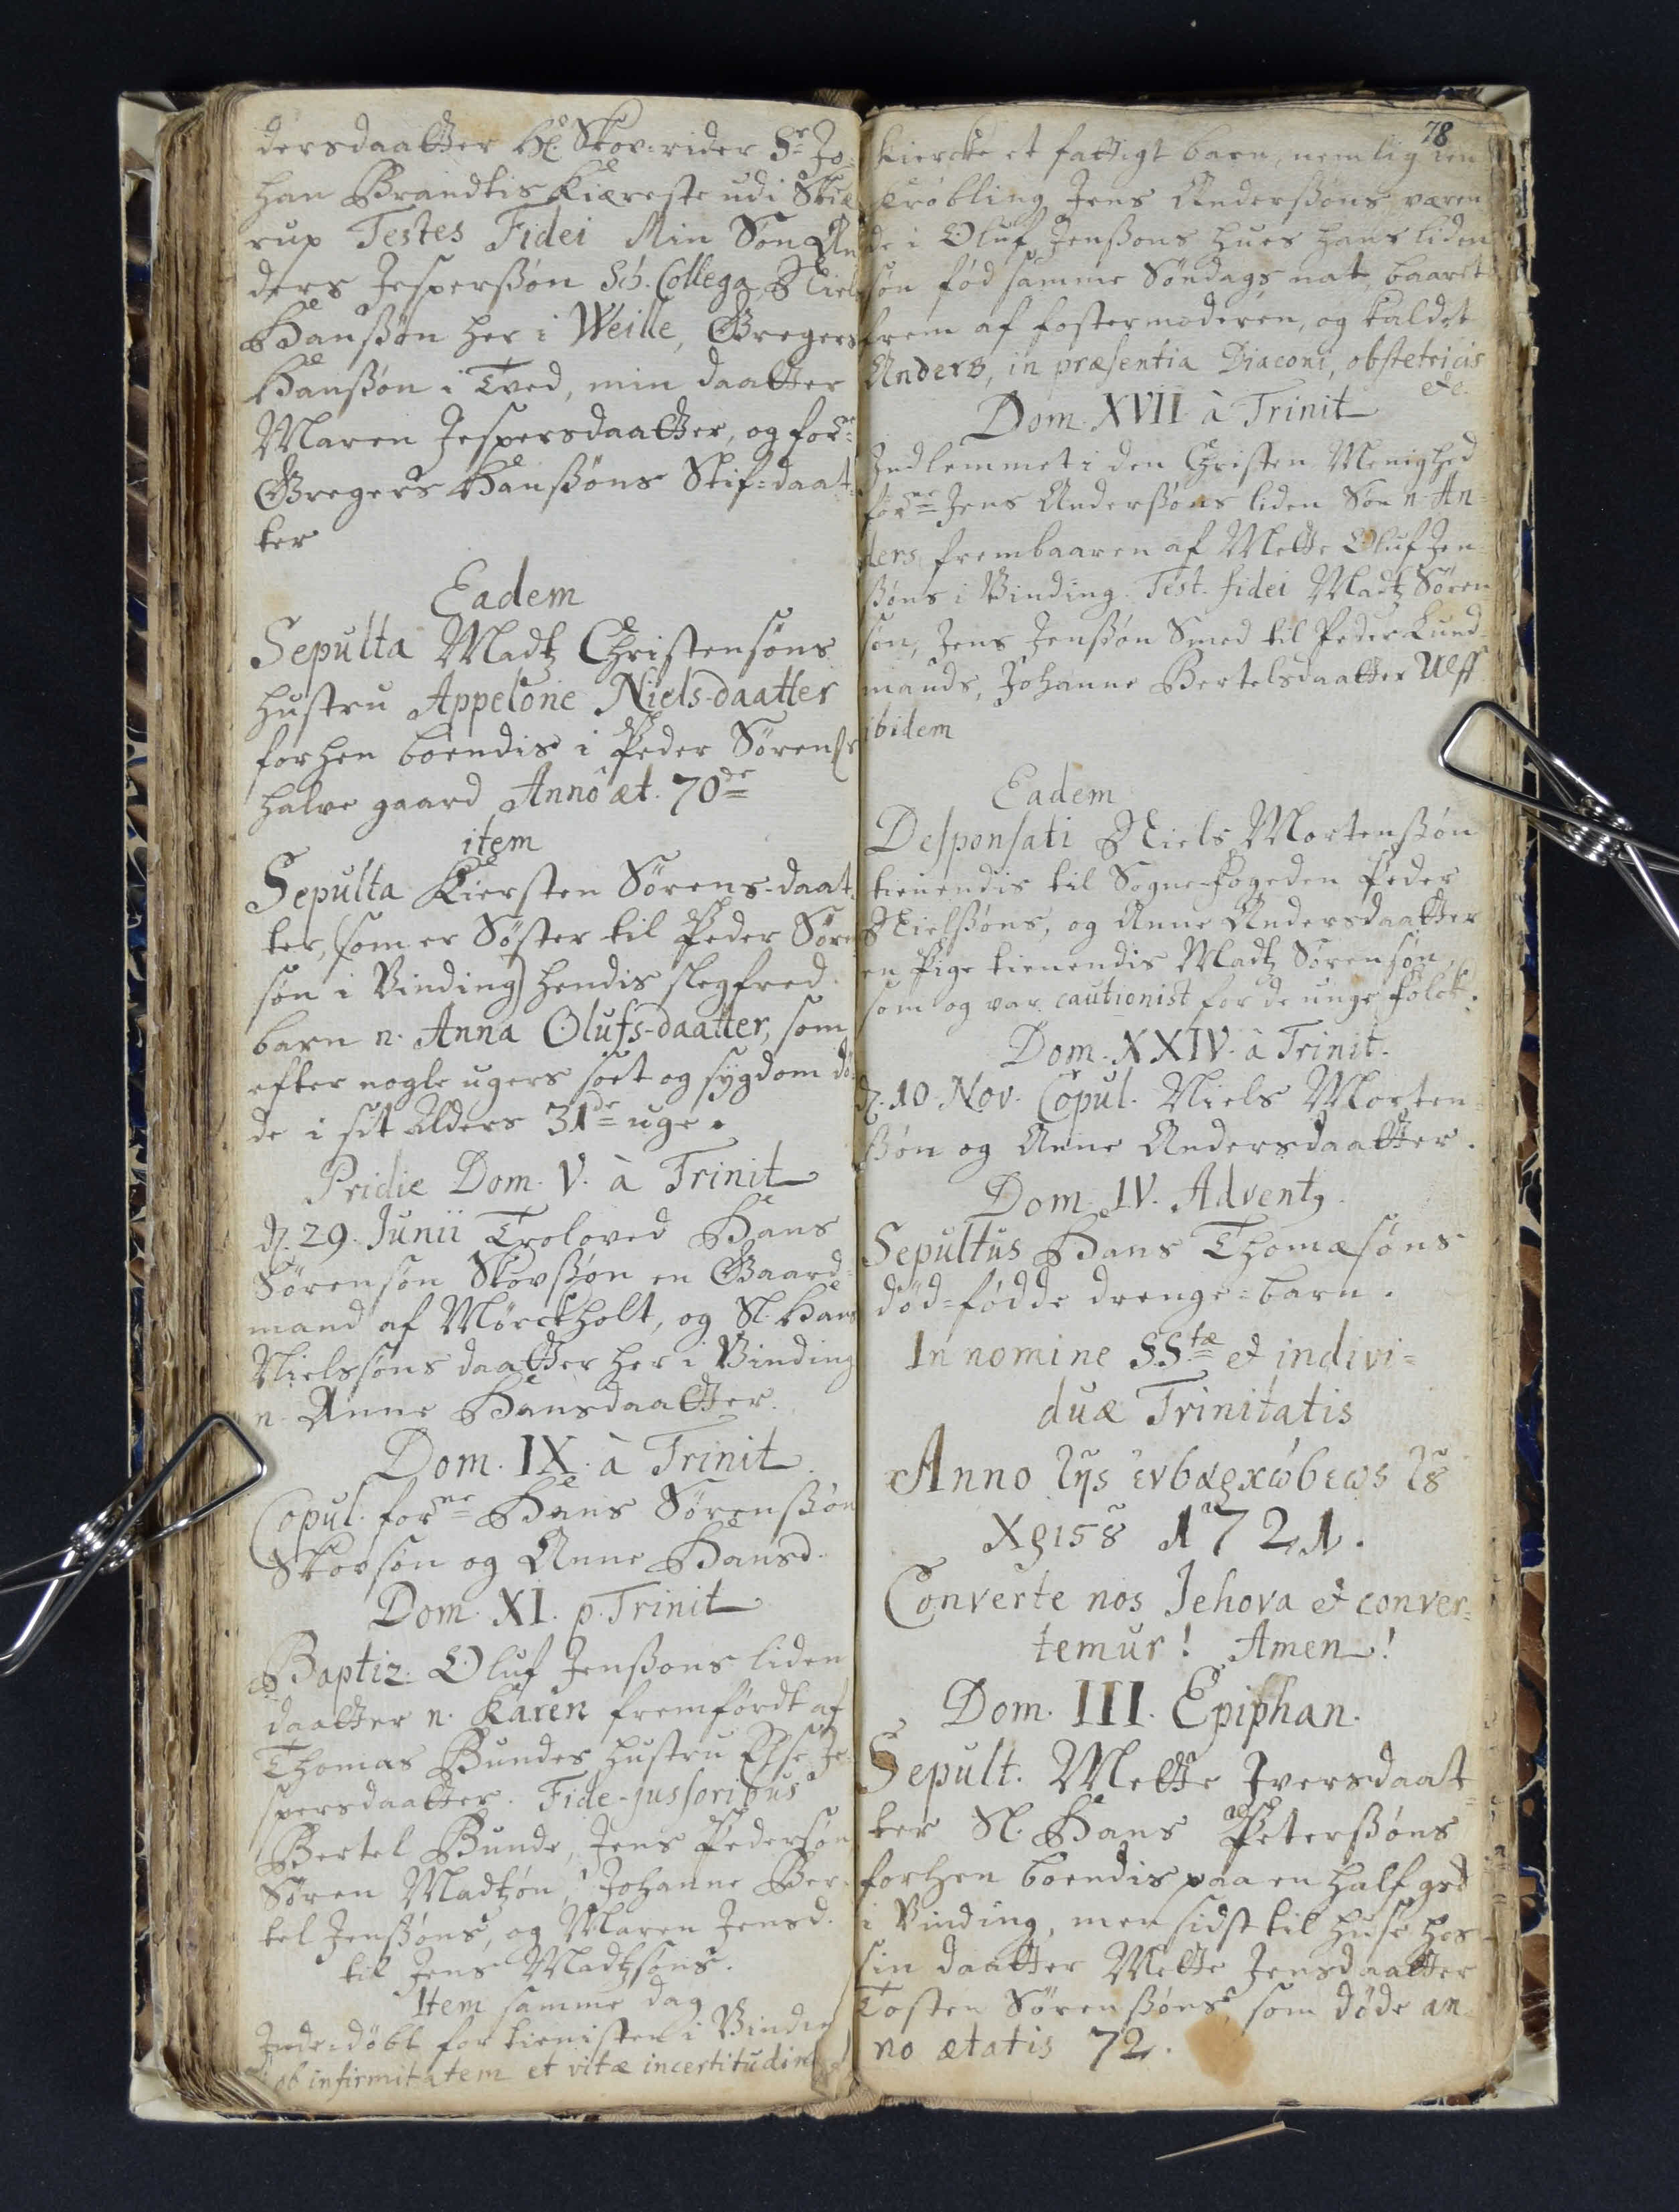dersdaatter Hl Skovrider Sr Jo¬
han Brandtis Kiæriste udi Skiæ
rup Testes Fidei Min Søn An
ders Jesperssøn Scb. Collega. Niels
Hanssøn her i Weille, Gregers
Hanssøn i Tved. min daatter
Maren Jespersdaatter, og forne
Gregers Hanssøns Stif=daat¬
ter
Eadem
Sepulta Madtz Christensøns
hustru Appelone Nielsdaatter 
forhen boendis i Peder Sørenss
halve gaard Anno æt. 70de
item
Sepulta Kiersten Sørens-daat¬
ter, (som er Søster til Peder Søre¬
søn i Vinding) hendis slegfred
barn n. Anna Olufs-daatter, som
efter nogle ugers soet og sygdom dø¬
de i sit Alders 31de uge.
Pridie Dom. V. a Trinit
d. 29. Junii Troloved Hans
Sørensøn Skovssøn en Gaard¬
mand af Mørckholt, og Sl. Hans
Nielsøns daatter her i Vinding
n. Anne Hansdaatter
Dom. IX. a Trinit
Copul. forne Hans Sørenssøn
Skovsøn og Anne Hansd.
Dom. XI. p. Trinit
Baptiz. Oluf Jenssøns liden
daatter n. Karen, fremførdt af
Thomas Bundes hustru Else Je¬
spersdaatter. Fide-jussoribus
Bertel Bunde. Jens Pedersøn
Søren Madtzøn, Johanne Ber¬
tel Jenssøns, og Maren Jensd.
til Jens Madtzsøns
Item samme dag 
Inde=døbt for tienesten i Vinding
## ob infirmitatem et vitæ incertitudi##
78
Kiercke, et fattigt barn, nemlig een
krøbling Jens Anderssøns væren
de i Oluf Jenssøns hues, hans liden
søn fød samme søndags nat, baaret
frem af foster moderen, og kaldet
Anders, in præsentia Diaconi obstetricis
etc.
Dom. XVII a Trinit
Indlemmet i den Christen Menighed
forne Jens Anderssøns liden Søn n. An¬
ders frembaaren af Mette Olufs Jen¬
ssøns i Vinding. Test. fidei Madtz Søren
søn, Jens Jenssøn Smed til Peder Lund¬
mands, Johanne Bertelsdaatter Ulff
ibidem
Eadem
Desponsati Niels Mortenssøn
tienendis til Sogne-fogeden Peder
Nielssøns, og Anne Andersdaatter
en Pige tienendis Madtz Sørensøn
som og var cautionist for de unge folck.
Dom. XXIV. a Trinit.
d. 10 Nov. Copul. Niels Morten¬
ssøn og Anne Andersdaatter
Dom. IV. Adventq
Sepultus Hans Thomæsøns 
død=fødde drenge=barn.
In nomine SStæ et indivi¬
duæ Trinitatis
Anno ##s evbxqx##be##s ##
Xqi## 1721
Converte nos Jehova et conver¬
temur! Aman !
Dom. III. Epiphan.
Sepult. Mette Iversdaat¬
ter Sl. Hans Peterssøns
forhen boendis paa en half grd
i Vinding, men sidst til huse hos 
sin daatter Mette Jensdaatter
Tosten Sørenssøns, som døde an¬
no ætatis 72